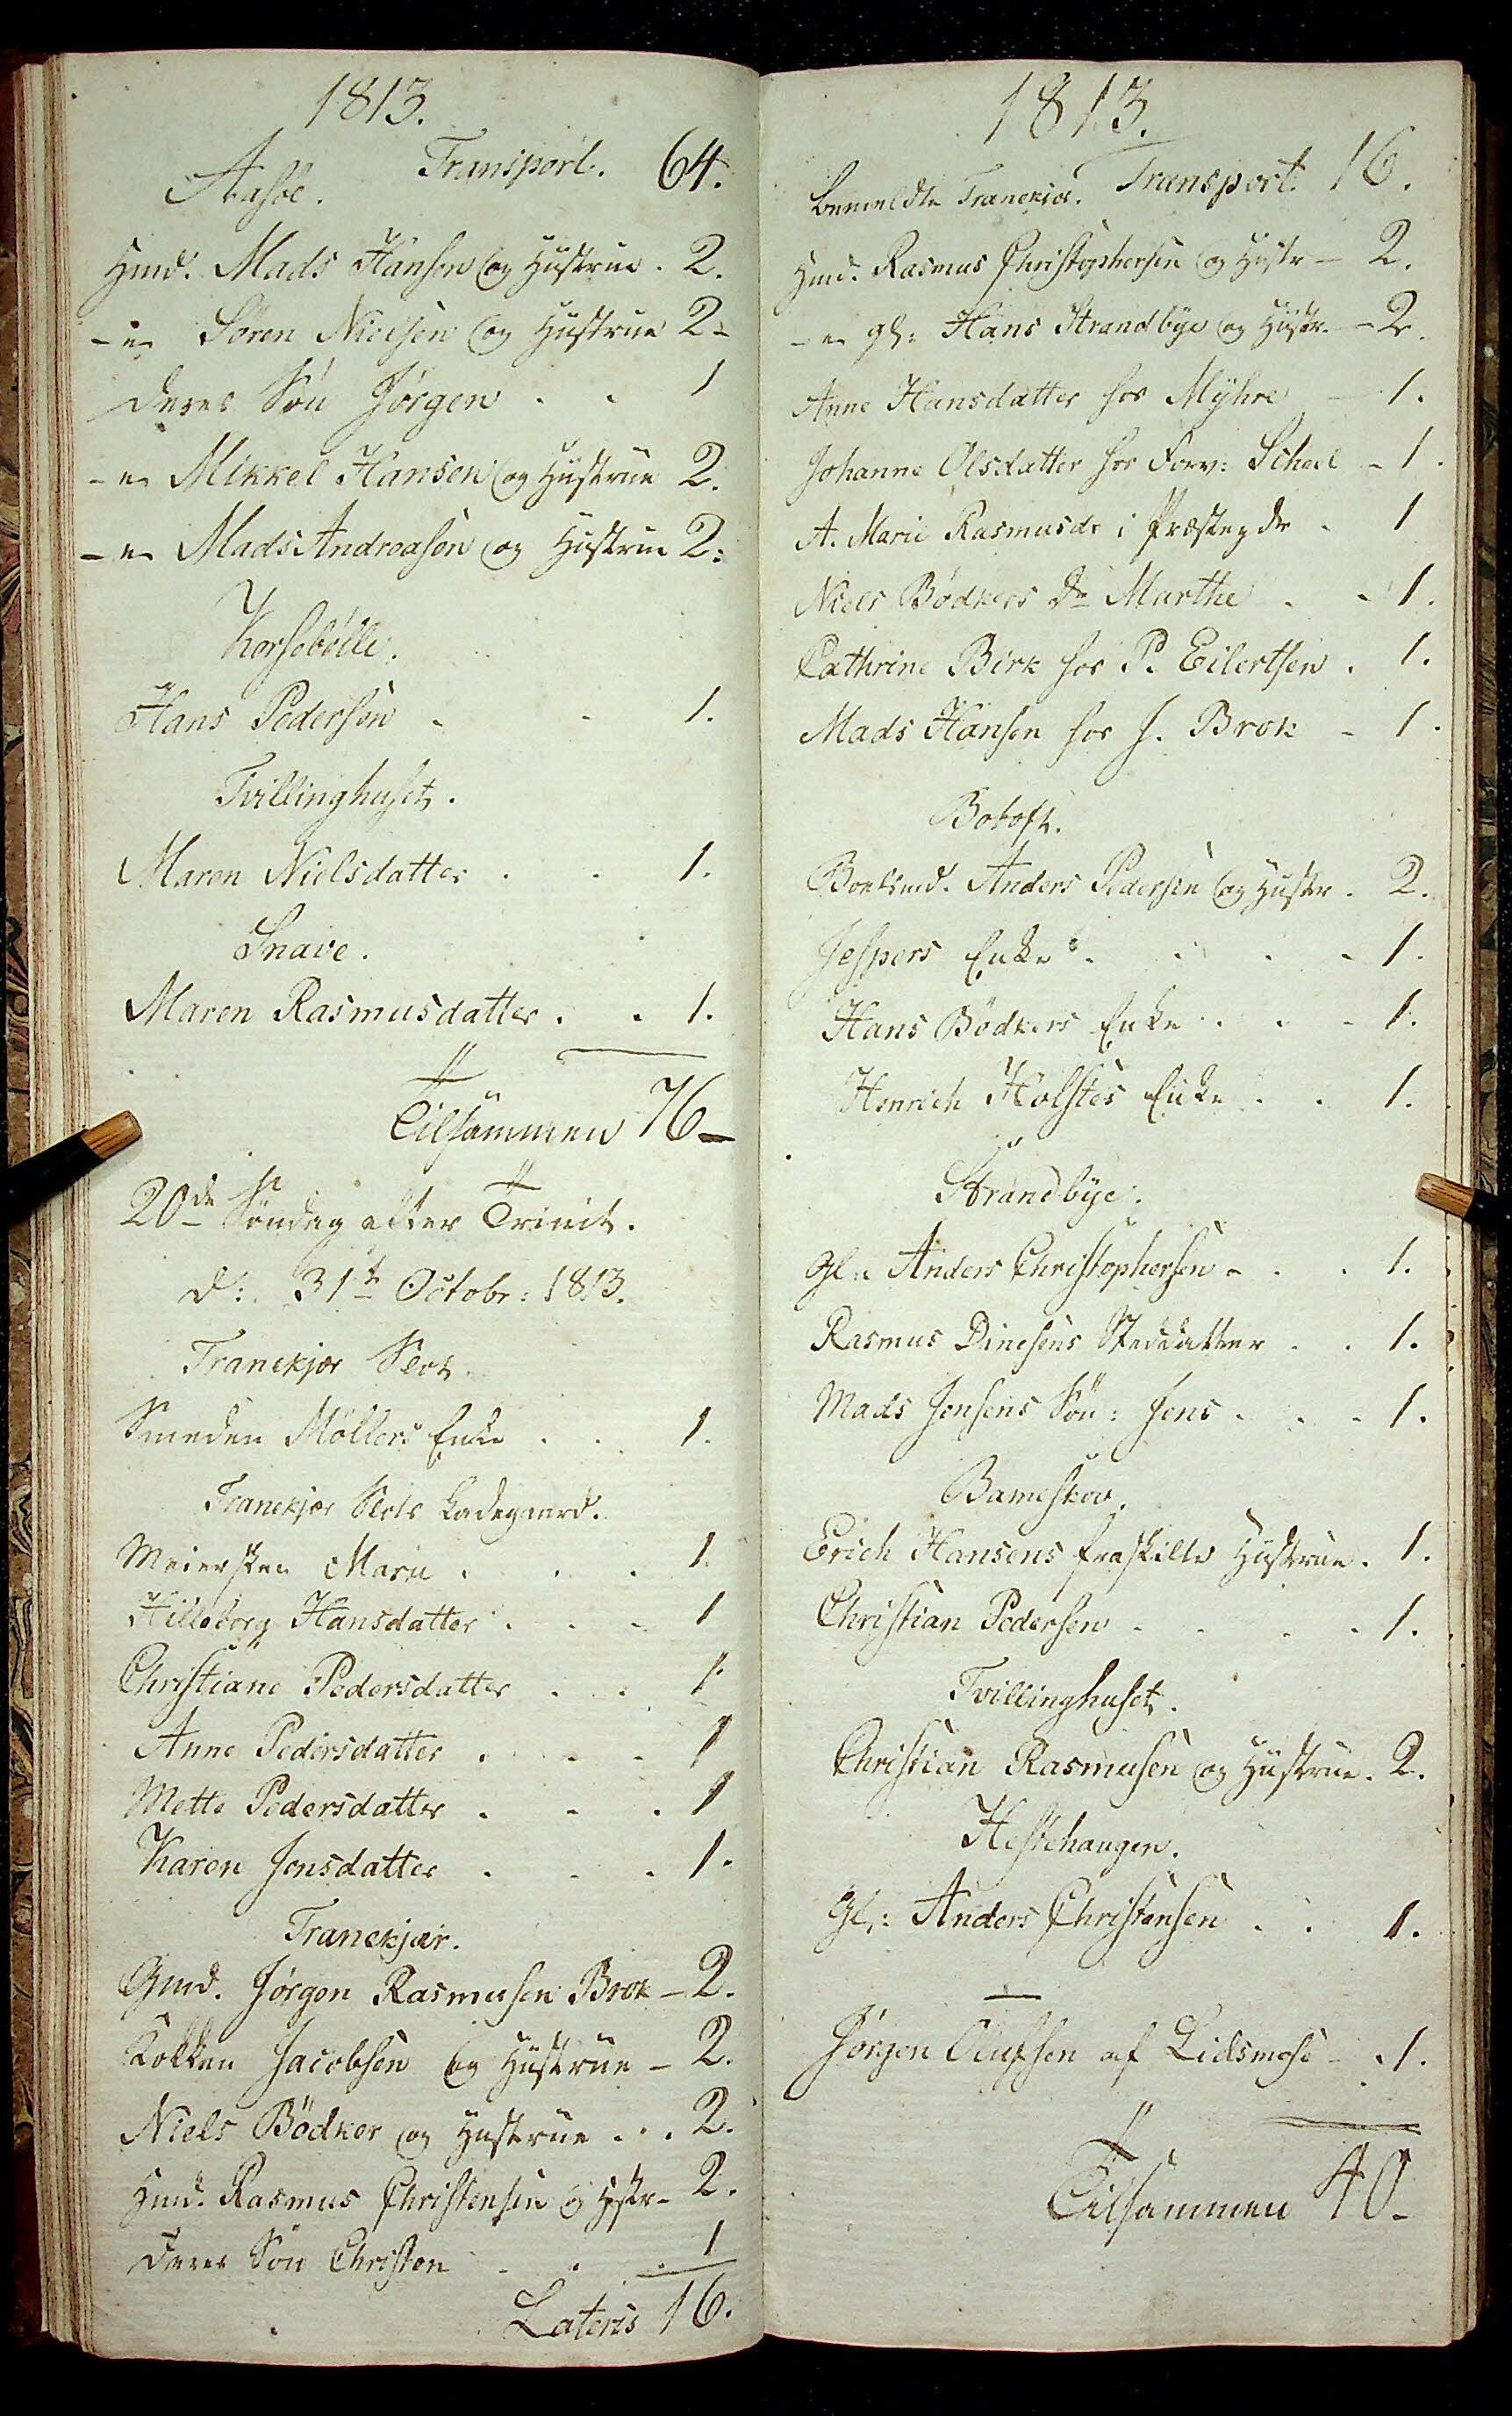1813.
Aasøe. Transport. 64.
Hmd. Mads Hansen og Hustrue . 2.
-"- Søren Nielsen og Hustrue 2-
deres Søn Jørgen . . 1
-"- Mikkel Hansen og Hustrue 2.
-"- Mads Andreasen og Hustrue 2:
Korsebølle.
Hans Pedersen . . 1.
Tvillinghuset.
Maren Nielsdatter . . 1.
Snave.
Maren Rasmusdatter . . 1.
Tilsammen 76-
20de Søndag efter Trinit.
d: 31te Octobr: 1813.
Tranekjær Slot
Smeden Møllers Enke . . 1.
Tranekjær Slots Ladegaard.
Miersken Marie . . . 1.
Hilleborg Hansdatter . . . 1
Christiane Pedersdatter . .1
Anne Pedersdatter . . . 1
Mette Pedersdatter . . . 1
Karen Jensdatter . . . 1
Tranekjær.
Gmd. Jørgen Rasmusen Brok - 2.
Kokken Jacobsen og Hustrue - 2.
Niels Bødker og Hustrue . . . 2.
Hmd. Rasmus Christensen og Hstr - 2.
deres Søn Christen . . . 1
Lateris 16.
1813.
bemeldte Tranekiær. Transport. 16.
Hmd. Rasmus Christophersen og Hustr - 2.
-"- gl: Hans Strandbye og Hustr . - 2
Anne Hansdatter hos Myhre - 1.
Johanne Olsdatter hos Forv: Scheel - 1
A. Marie Rasmusdr i Præstegdr . 1
Niels Bødkers Dr Marthe . . 1
Cathrine Birk hos P. Eilertsen . 1.
Mads Hansen hos J. Brok - 1
Botoft.
Boelsmd. Anders Pedersen og Hustr. 2.
Jespers Enke . . . . 1.
Hans Bødkers Enke . . . 1.
Henrich Holstes Enke . . 1.
Strandbye.
Gl: Anders Christophersen - . .1.
Rasmus Dinesens Steddatter . . 1.
Mads Jensens Søn: Jens . . . 1.
Bameskov
Erich Hansens fraskilte Hustrue . 1.
Christian Pedersen . . . .  1.
Tvillinghuset.
Christian Rasmusen og Hustrue . 2.
Hestehaugen.
Gl: Anders Christensen . . 1.
Jørgen Olufsen af Lidsmose - . 1.
Tilsammen 40.
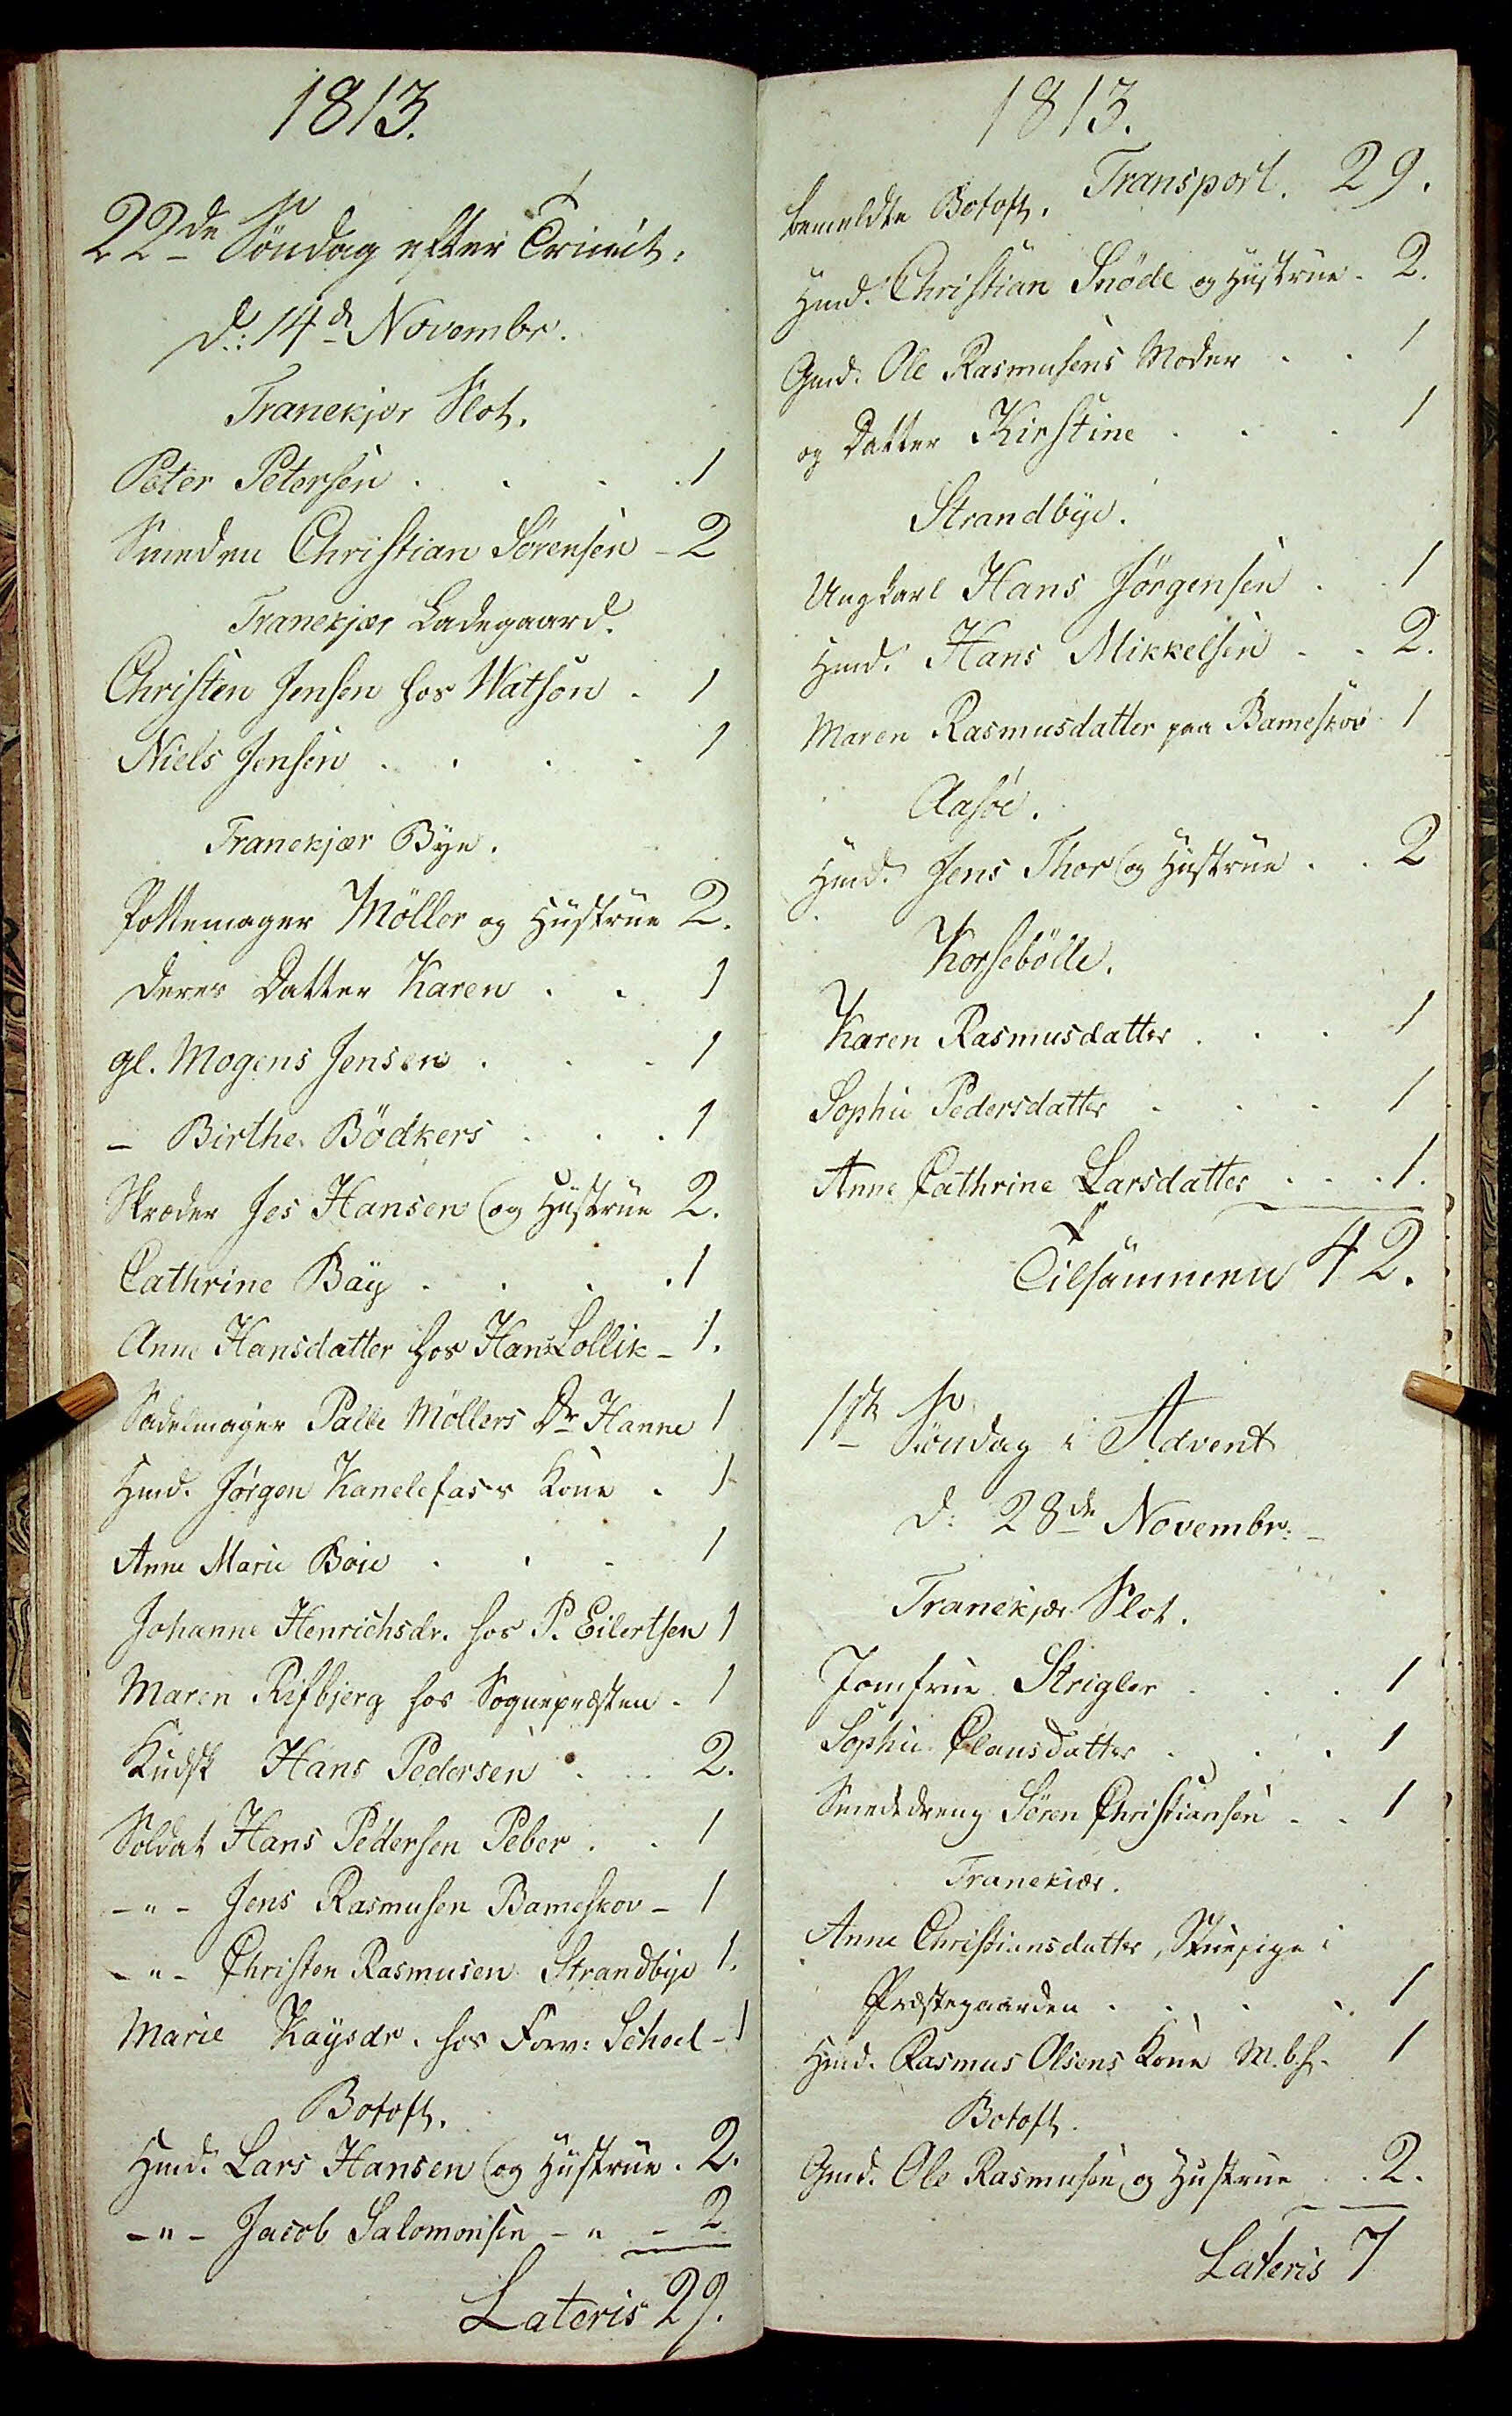1813.
22de Søndag efter Trinit.
d: 14de Novembr.
Tranekjær Slot.
Peter Petersen . . . . 1
Smeden Christian Sørensen - 2
Tranekjær Ladegaard.
Christen Jensen hos Watson . 1
Niels Jensen . . . . 1
Tranekjær Bye.
Pottemager Møller og Hustrue 2.
deres Datter Karen . . 1
gl. Mogens Jensen . . . 1
- Birthe Bødkers . . . 1
Skræder Jes Hansen og Hustrue 2.
Cathrine Bay . . . . 1
Anne Hansdatter hos Hans Lollik - 1.
Sadelmager Palle Møllers Dr Hanne 1
Hmd. Jørgen Kanelefass Kone . 1
Anne Marie Bois . . . 1
 Johanne Henrichsdr. hos P. Eilertsen 1
Maren Rifbjerg hos Sognepræsten . 1
Kudsk Hans Pedersen . . . 2.
Soldat Hans Pedersen Peber . . 1
-"- Jens Rasmusen Bameskov - 1
-"- Christen Rasmusøn Srrandbye 1.
Marie Kaysdr. hos Forv: Scheel - 1
Botoft.
Hmd. Lars Hansen og Hustrue . 2.
-"- Jacob Salomonsen -"- 2.
Lateris 29.
1813.
bemeldte Botoft. Transport. 29.
Hmd. Christian Snøde og Hustrue . 2.
Gmd. Ole Rasmusens Moder . . 1
og Datter Kirstine . . . 1
Strandbye.
Ungkarl Hans Jørgensen . . 1
Hmd. Hans Mikkelsen . .  2.
Maren Rasmusdatter paa Baameskov
Aasøe.
Hmd. Jens Thor og Hustrue . . 2
Korsebølle.
Karen Rasmusdatter . . . 1
Sophie Pedersdatter . . . 1
Anne Cathrine Larsdatter . . . 1.
Tilsammen 42.
1ste Søndag i Advent
d: 28de Novembr:
Tranekjær Slot
Jomfrue Strigler . . . 1
Sophie Clausdatter . . . 1
Smedrenge Søren Christiansøn . . 1
Tranekiær.
Anne Christiansdatter, Stuepige i
Præstegaarden . . . . 1
Hmd. Rasmus Olsens Kone M.b.s. 1
Botoft.
Gmd. Ole Rasmusen og Hustrue . . 2.
Lateris 7